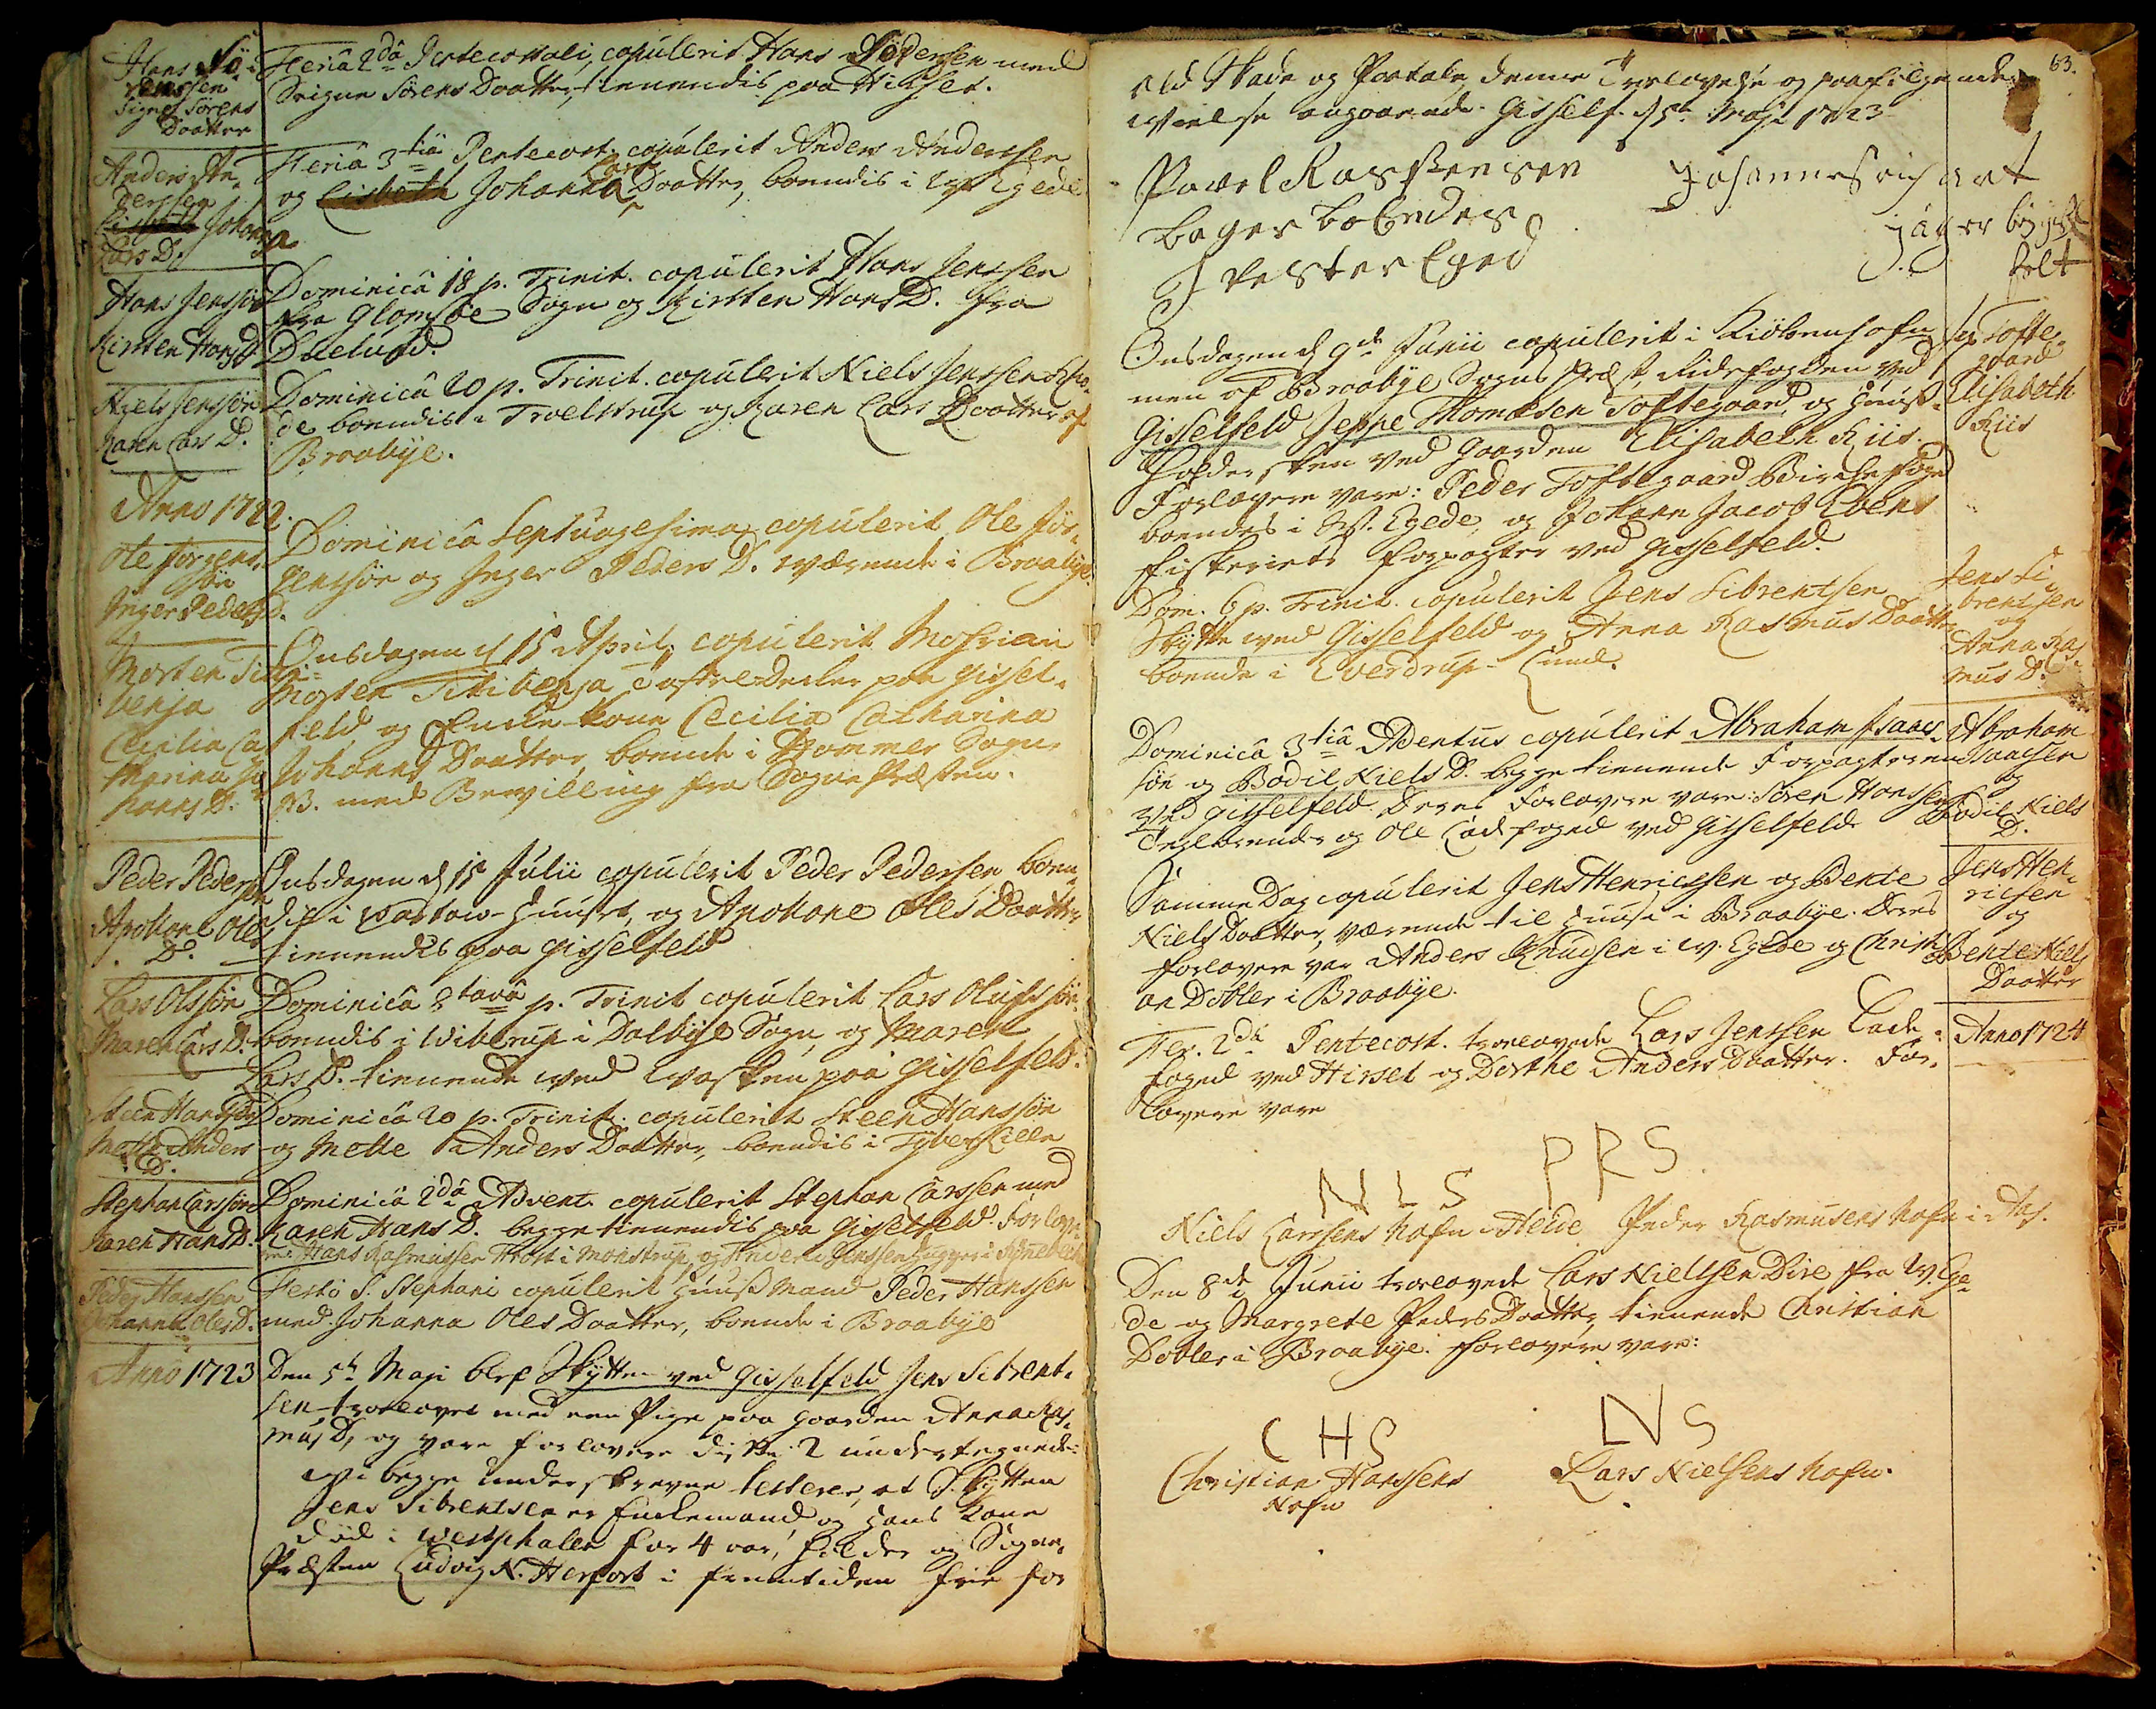Hans Sø¬
rensen 
Signe Sørens
Datter 
Feria 2da Pentecosti## copulerit Hans Sørensen## med
Seigne Sørens Daatter, tiendendis paa Hirset. 
Andes An¬
derssen 
Lisbeth Johanna
Lars D.
Feria 3tia Pentecost. copulerit Anders Anderssen
Lars
og Lisbeth Johanna Daatter, boendis i W. Egede.
Hans Jenssøn
Kirsten HansD. 
Dominica 18 p. Trinit. copulerit Hans Jenssen
fra Glomsøe Sogn og Kirsten HansD. fra
Duelund.
Niels Jensen 
Karen LarsD.
Dominica 20 p, Trinit. copulerit Niels Jensen der##¬
de boendis i Troelstrup og Karen Lars Daatter af 
Braabye.
Anno 1722
Ole Jørgens
søn
Inger PedersD.
Dominica Septuagesima copulerit Ole Jør¬
gensøn og Inger PedersD. værende i Braabye.
Morten Tili¬
benja 
Cecilia Ca
tharina Jo
hannsD.
Onsdagen d. 15 April copulerit Mohrian 
Morten Titibenja Taffeldecker paa Gissel¬
feld og Enche-kone Cecilia Catharina
Johanns Daatter boende i Hammer Sogn.
NB. med bevilling fra Sogne Præsten.
Peder Pedersen
Apollone Oles
D. 
Onsdagen d. 15 July copulerit Peder Pedersen boen¬
dis i Wartov Huuset, og Apollone Oles Daatter 
tienendis paa Gisselfeld. 
Lars Olssøn 
Maren LarsD.
Dominica 8tava p.Trinit. copulerit Lars Olufssøn
boendis i Wiberup i Dalbye Sogn, og Maren
Lars D. tienende ved Vasken paa Gisselfeld.
Steen Hansøn 
Mette Anders
D. 
Dominica 20 p. Trinit. copulerit Steen Hanssøn
og Mette Anders Daatter, boendis i TybergLille.
Stephan Larsøn 
Karen HansD.
Dominica 2da Advent copulerit Stephan Larssen med
Karen HansD. begge tienendis paa Gisselfeld. Forlove-
re Hans Rasmussen Frost## i Monstrup og Frideric Hansen## Hugger i Rønnebeck
Peder Hansen
Johanne OlesD.
Festo St. Stephani copulerit HuusMand Peder Hanssen
med Johanne Oles Daatter, boende i Braabye.
Anno 1723 
Dom 5ta May blef Skytten ved Gisselfeld Jens Sibrent¬
sen trolovet med eén Pige paa Gaarden Anna Ras¬
musD. og være forlover disse 2 undertegnende:
ere begge underskrevne testerer, at Skytten 
Jens Sibrentsen er Enkemand, og hans kone
døde i Westphalen for 4 aar, holder## og Sogne¬
Præsten Ludvig N.Herfort i fremtiden Frie for 
ald Skade og Paatale denne Trolovelse og påfølgende
Vielse angaaende Gisself. d. 5 May 1723
Povel Rasskensen##
Johannesøn##
Bager boendes
Jæger ## byg##
i Wester Eged
holt##
53.
Onsdagen d. 9de Junii copulerit i Kiøbenhafn
men af Braabye Sogns Præst, Ridefogden ved
Gisselfeld Jeppe Thomasen Toftegaard og Huus¬
holdersken ved Gaarden Elisabeth Riis
Forlovere vare: Peder Toftegaard Birkefoged
boendis i V. Egede, og Johan Jacob Evens
Fiskeriets Forpagter ved Gisselfeld.
Jep Tofte¬
gaaard 
Elisabeth 
Riis 
Dom 6 p. Trinit. copulerit Jens Sibrentsen 
Skytte ved Gisselfeld og Anna Rasmus Daatter 
boende i Everdrup Lund. 
Jens Si
brentsen
og 
Anna Ras
musD.
Dominica 3tia Adventus copulerit Abraham Isaacs¬
søn og Bodil NielsD. begge tiendende Forpagteren 
ved Gisselfeld. Deres Forlovere vare: Søren Hanssen
Teglbrender og Ole Ladfoged ved Gisselfeld.
Abraham 
Isaacssøn
og 
Bodil Niels
D.
Samme Dag copulerit Jens Henrihcsen og Bente
Nields daatter, værende til huuse i Braabye. Deres
forlovere var Anders Knudsen i W. Egede og Christi
an Dobler i Braabye.
Jens Hen¬
ricsen 
og 
Bente Niels
Datter
Fer. 2da Pentecost. troelovede Lars Jenssen Lade¬
foged ved Hirset og Dorthe Anders Daatter. For¬
lovere vare 
P R S
N L S
Niels Larssens Nafn i Heide## Peder Rasmusens Nafn i Aas.
Anno 1724
Den 8de Juny troelovede Lars Nielssen Dire fra W. Ege¬
de og Margrete Peders Daatter, tienende Christian
Dobler i Braabye. Forlovere vare:
L V S
C H S
Lars Nielsens Nafn
Christian Hanssens
Nafn
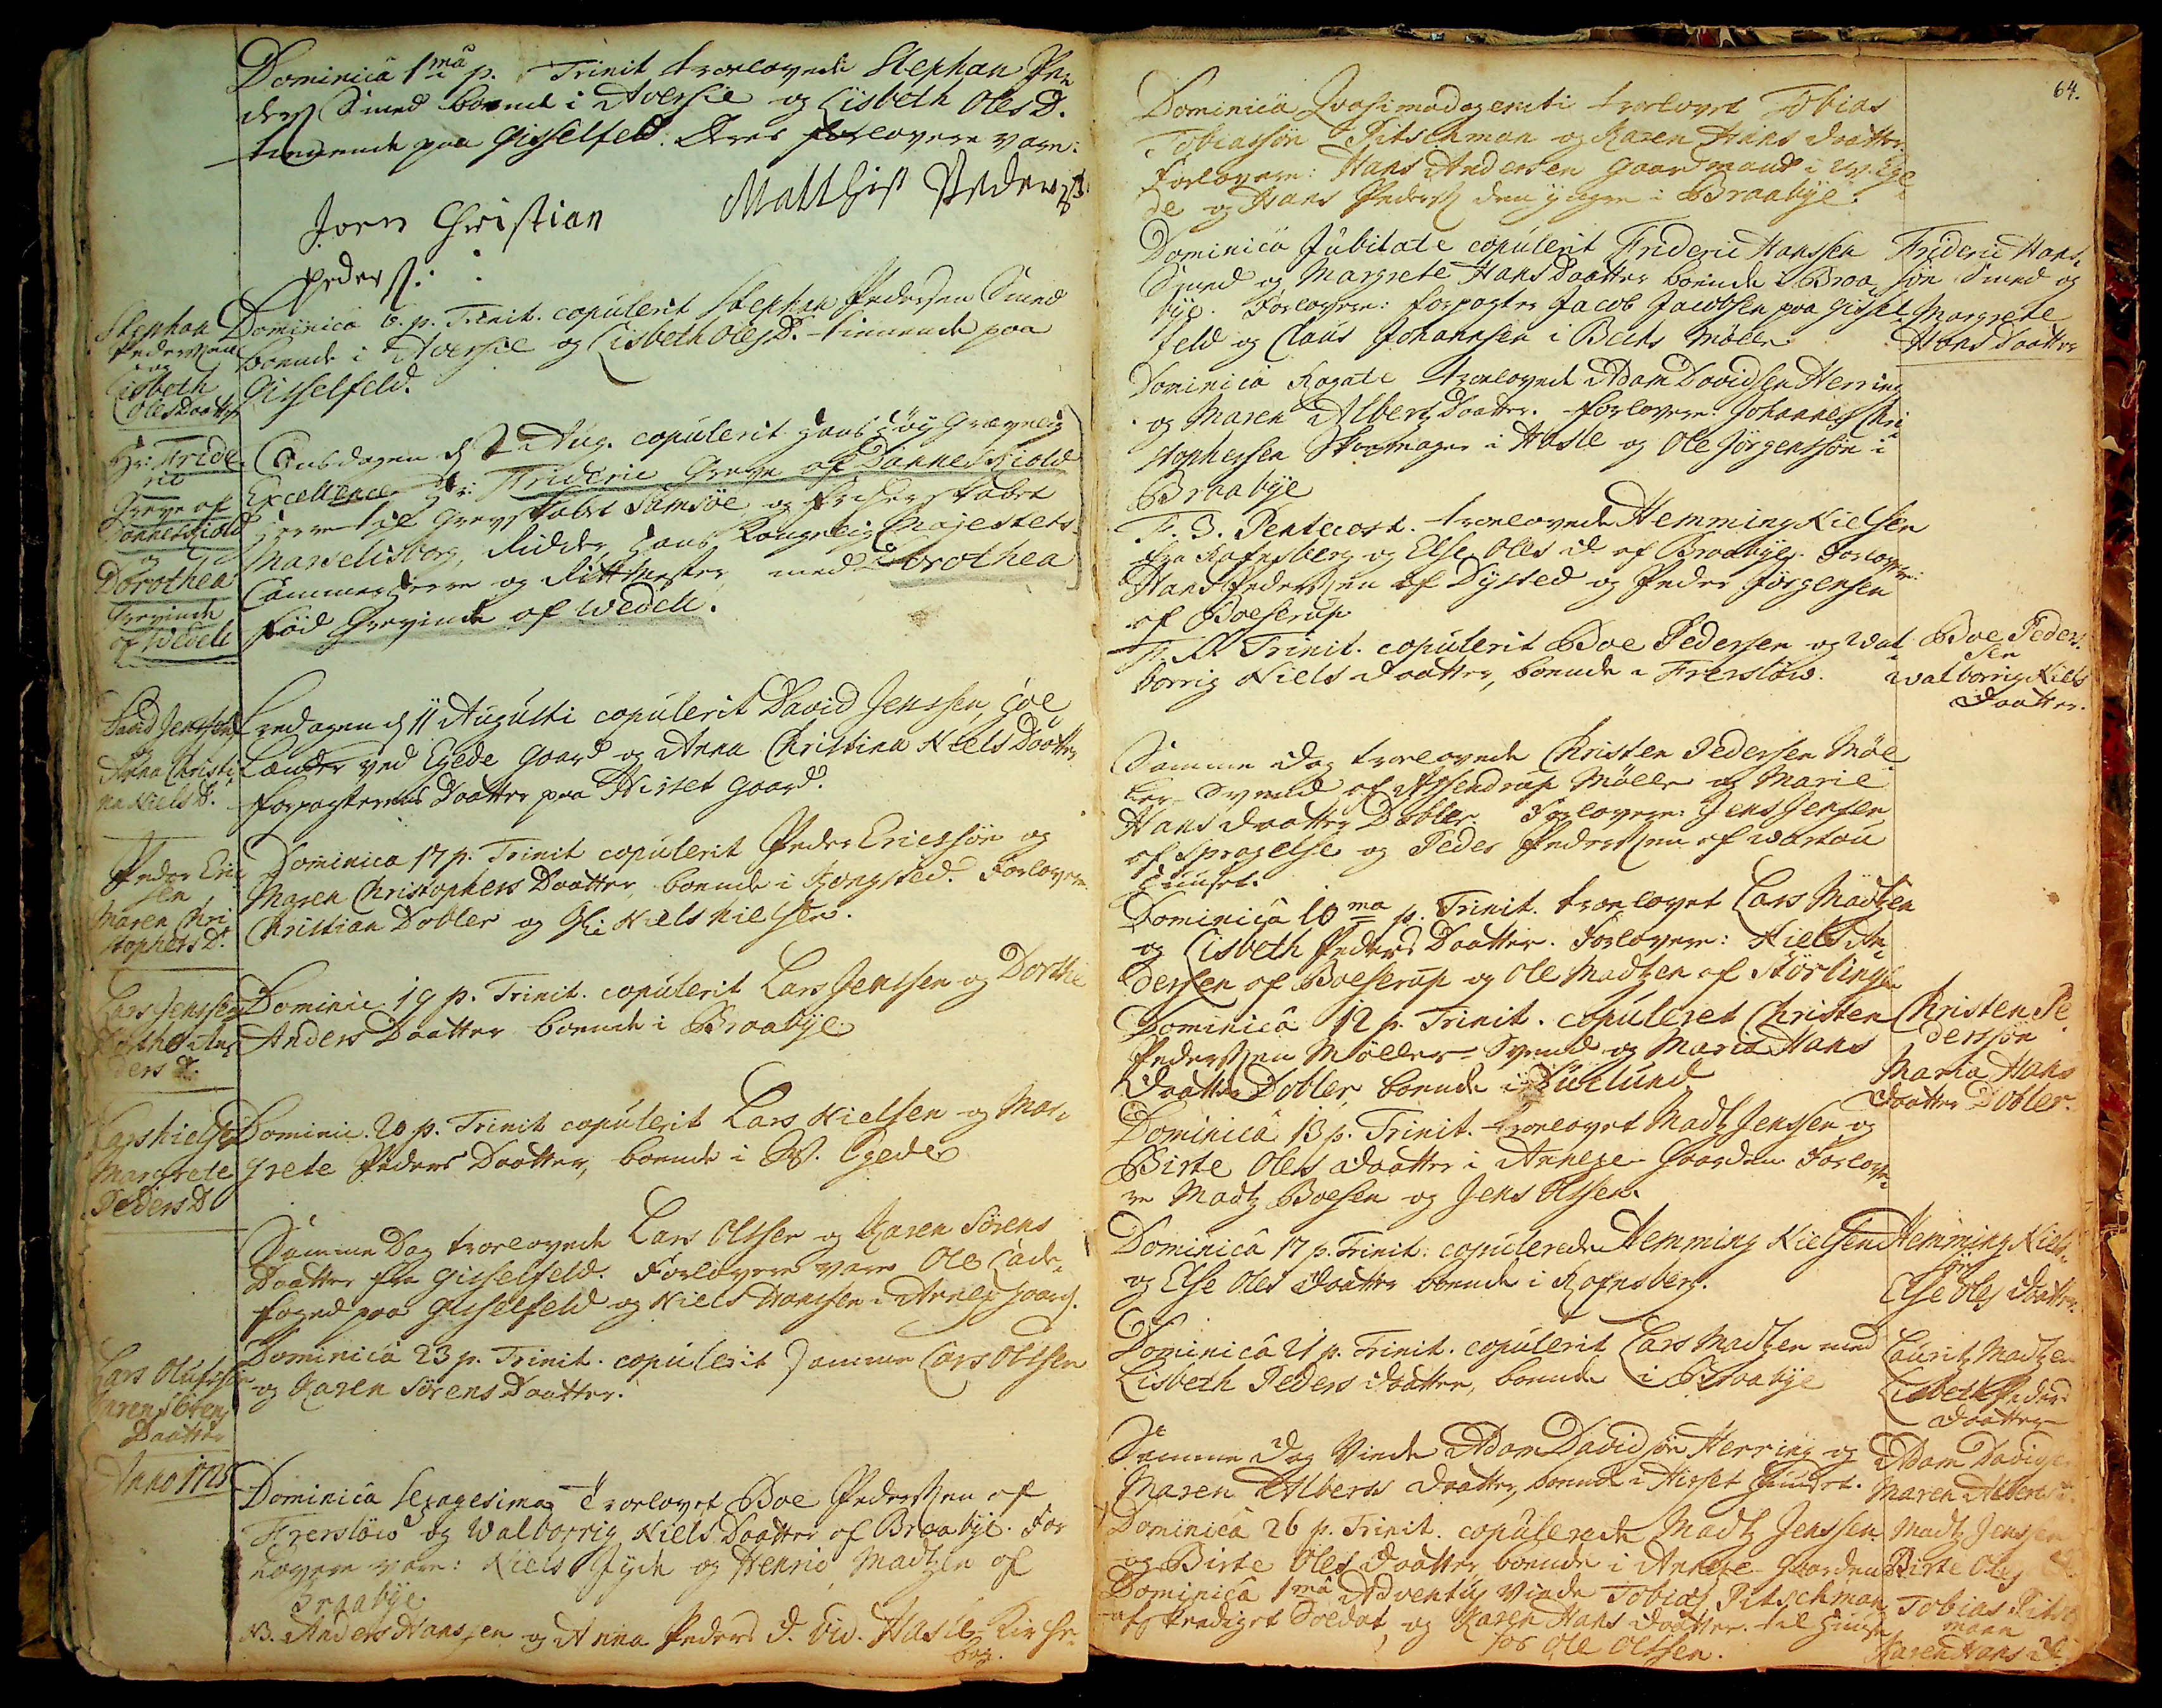Dominica 1ma p. Trinit. trolovede Stephan Pe¬
ders Smed boende i Aversie og Lisbeth OlesD. 
tienende paa Gisselfeld. Deres forlovere vare:
Joen Christian
Matthiss Pedersøn
Peders:
Stephen
Pedersen
og
Lisbeth
OlesDaatter
Dominica 6 p. Trinit. copulerit Stephen Pedersen Smed
boende i Aversie og Lisbeth OlesD. - tienende paa
Gisselfeld.
Hr. Fride¬
ric
Greve af
Danneskiold
Dorothea
Grevinde
af Wedell
Onsdagen d. 2. Aug. Copulerit Hans Høÿ Grævelig
Execellence Si: Frideric Greve af Danneskiold
Herre til Grevskabet Samsøe og Friherskabet
Marselisborg, Ridder, Hans Kongelig Majestets
Cammerherre og Rittmester med Dorothea
fød Grevinde af Wedell.
David Jensen 
Anna Christi¬
na NielsD.
Fredagen d. 11 Augusti copulerit David Jensen, Hol¬
lænder ved Egede Gaard og Anna Christina Niels Daatter  
Forpagterrens Daatter paa Hirset gaard.
Peder Eric
sen
Maren Chri
stophersD.
Dominica 17 p. Trinit. copulerit Peder Ericssøn og
Maren Christophers Daatter, boende i Kongsted, Forlovere
Christian Dobler og Gl: Niels Nielsen. 
Lars Jensen 
Dorthe An¬
dersD. 
Dominica 19 p. Trinit. copulerit Lars Jenssen og Dorthe
Anders Daatter boende i Braabye.
Lars Nielsen 
Margrete
PedersD. 
Dominica 20 p. Trinit. copulerit Lars Nielsen og Mar-
grete Peders Daatter, boende i W. Egede. 
Samme Dag troloved Lars Olssen og Karen Sørens 
Daatter fra Gisselfeld. Forlovere vare Ole Lade-
foged paa Gisselfeld og Niels Hanssen i Annexgaarden.
Lars Olufssen
Karen Sørens
Daatter
Dominica 23 p. Trinit. copulerit samme Lars Olssen
og Karen Sørens Daatter. 
Anno 1725
Dominica Sexagesima trolovet Boe Pederssen af
Frersløv og Walborrig Niels Daatter af Braabye For
lovere Vare: Niels Jyde og Henric Madtzen af
Braabye. 
NB: Anders Hansen og Anna PedersD. vid. Hasle Kirche
Bog 
Dominica Quasimodo geniti trolovet Tobias
Tobiassøn Pitschman og Karen Hans Daatter.
Forlovere: Hans Andersen Gaardmand i W. Ege¬
de og Hans Peders den Yngre i Braabye:
64.
Dominica Jubilate copulerit Frideric Hanssen
Smed og Margrete Hans Daatter i boende i Braa¬
bye. Forlovere: Forpagter Jacob Jacobsen paa Gissel¬
feld og Claus Johannsen i Becks Mølle.
Frideric Han¬
søn Smed og 
Margrete 
HansDaatter.
Dominica 21 p. Trinit. copulerit Lars Madtzen med
Lisbeth Peders Daatter, boende i Braabye.
Lauritz Madtzen
Lisbeth Peders
Daatter
Samme Dag Viede Adam Davidsøn Herring og
Maren Alberts Daatter, boende i Hirset Huuset.
Adam Davidsen
Maren AlbertsD.
Dominica Rogate troelovet Adam Davidsen Herring
og Maren Alberts Daatter. Forlovere: Johannes Chri¬
stophersen Skomager i Hasle og Ole Jørgenssøn i
Braabye.
F3. Pentecost. troeloeved Hemming Nielsen
fra Rafnsberg og Else OlesD. af Braabye. Forlovere:
Hans Pederssen af Dysted og Peder Jørgensen
af Boeserup.
F.SS. Trinit. copulerit Boe Pedersen og Wal¬
borrig Niels Daatter, boende i Frersløv. 
Boe Peder
sen
Walborrig Niels
Daatter 
Samme dag troeloved Christen Pedersen Møl¬
ler Svend af Assendrup Mølle og Marie
Hans Daatter Dobler. Forlovere: Jens Jensen
af Spragelse og Peder Pederssen af Wartou
Huuset. 
Dominica 10ma p. Trinit. troelovet Lars Madtzen
og Lisbeth Peders Daatter. Forlovere: Niels An¬
dersen af Boeserup og Ole Madtzen af Størlinge.
Dominica 12 p. Trinit. copuleret Christen
Pederssen Møller- Svend og Maria Hans
Daatter Dobler, boende i Duelund.
Christen Pe¬
dersøn
Maria Hans
Daatter Dobler
Dominica 13 p. Trinit. troelovet Madtz Jenssen og
Birte Oles Daatter i Annexe Gaarden. Forlove¬
re: Madtz Boesen og Jens Olssen. 
Dominica 17 p. Trinit. copulerede Hemming Nielsen
og Else Oles Daatter, boende i Rafnsberg.
Hemming Niels¬
søn
Else Oles Daatter
Dominica 26 p. Trinit. copulered Madtz Jenssen
og Birte Oles Daatter, boende i Annexe Gaarden.
Madtz Jensen 
Birte Oles
Dominica 1ma Adventus Viede Tobias Pitschman
afskradiget## Soldat og Karen Hans Daatter til Huuse
hos Ole Olssen.
Tobias Pits##
mann 
Karen HansD.